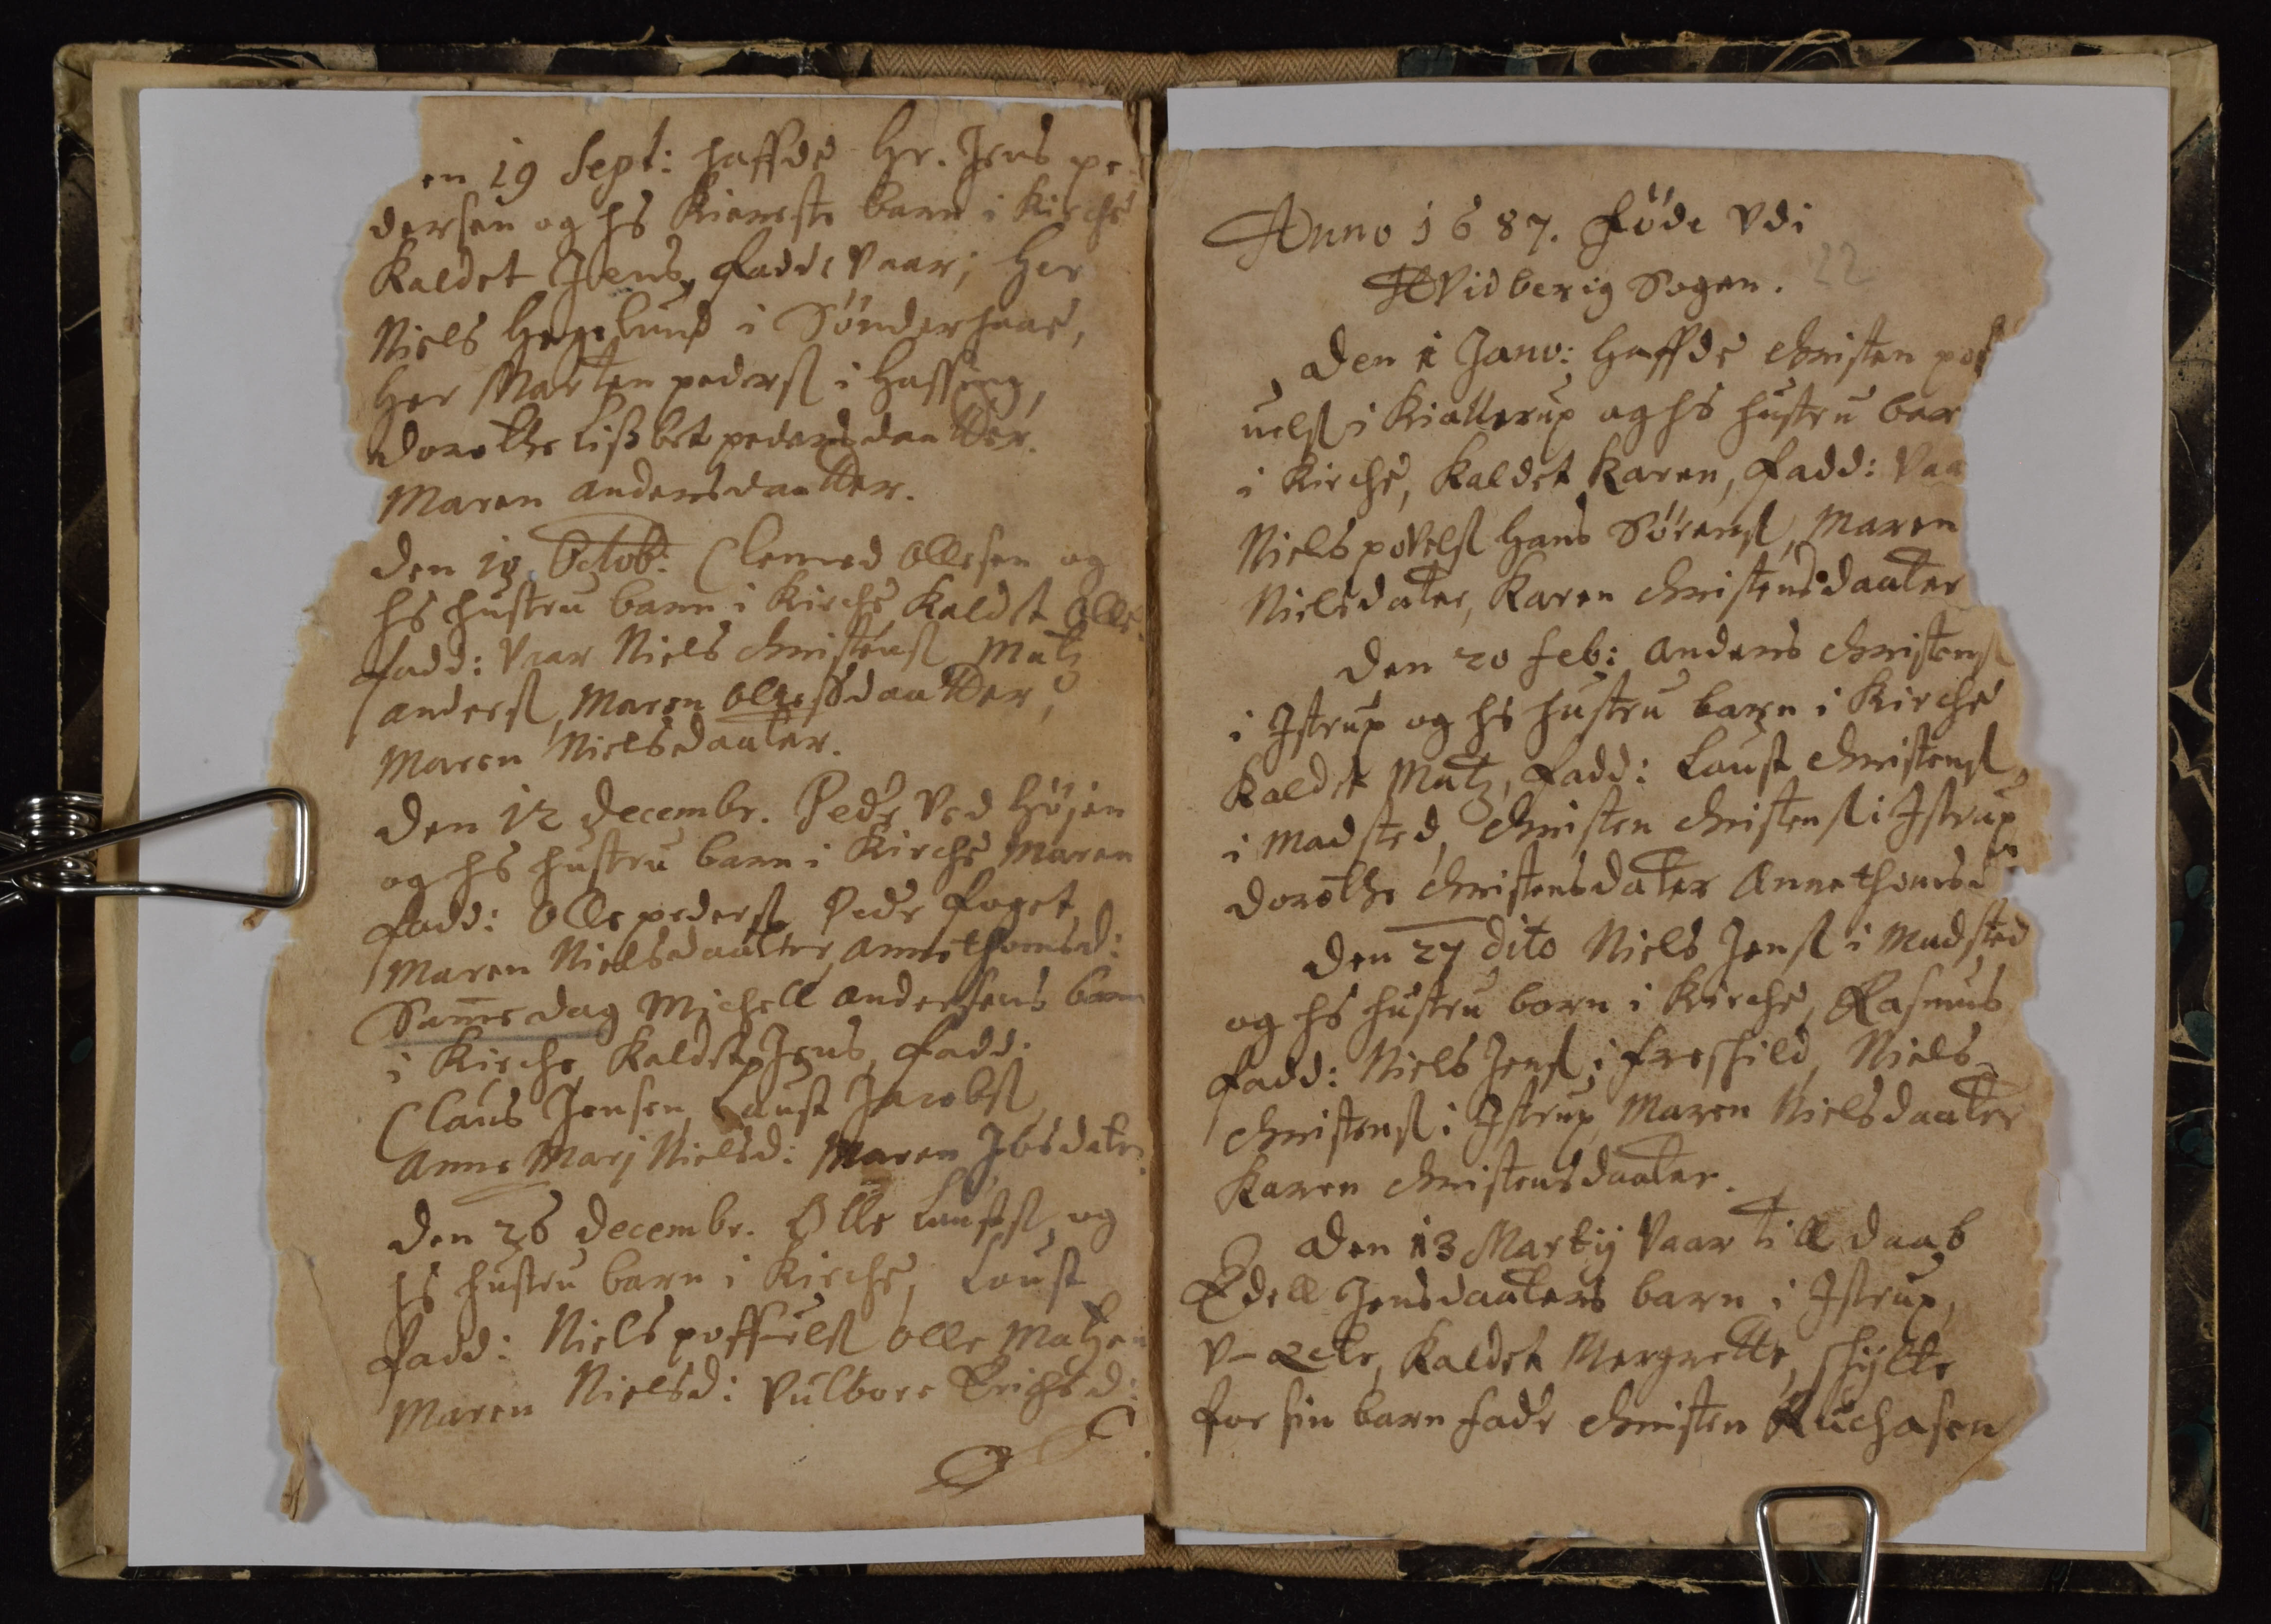en 19 Sept: haffde Hr. Jens pe¬
dersen og hs Kiæreste barn i Kirche
kaldet Jens, Fadd: vaar; Her
Niels Hegelund i Sønderhaae,
Her Marthen Peders i Hassing,
Dorothe Lissbet Pedersdaatter
Maren Andersdaatter
Den 19 Octob: Clemed Ollesen og
hs hustrue barn i Kirche kaldet Olle
Fadd: vaar Niels christens Matz
Anders Maren Ollessdautter,
Maren Nielsdauter.
Den 12 Decembr. Pedr Ved Højen
og hs hustru barn i Kirche Maren
Fsdd: Olle Peders Pedr Foget
Maren Nielsdautter Anne thomsd:
Samme dag Michell Andersens barn
i Kirche kaldet Jens, Fadd.
Claus Jensen Laust Jacobs
Anne Marj Nielsd: Maren Ibsdater
Den 26 Decembr. Olle Lausts og
hs hustru barn i Kirche, Laust
Fadd: Niels poffuels Olle Matzen
Maren Nielsd: Vulbore Erichsd:
Anno 1687. Føde vdi
Hvidberig Sogen.
Den 1 Janv: Haffde Christen pof
uels i Kiallerup og hs hustru baar
i Kirche, kaldet Karen, Fadde: vaa
Niels povels Hans Sørens, Maren
Nielsdater, Karen Christensdaater
Den 20 Feb: Anders Christens
i Istrup og hs hustru baren i Kirche
kaldet Matz, Fadd: Laust christens
i Madsted, Christen Christens i Istrup
Dorothe Christensdater Anne thomsd
den 27 dito Niels Jens i Madsted
og hs hustru barn i Kirche, Rasmus
Fadd: Niels Jens i Freshild, Niels
Christens i Istrup, Maren Nielsdaater
Karen christensdaater.
Den 13 Martijy vaar till daab
Edell Jensdaaters barn i Istrup.
V-æcte, kaldet Margrette, shylte
for sin barn fadr christen Luchasen
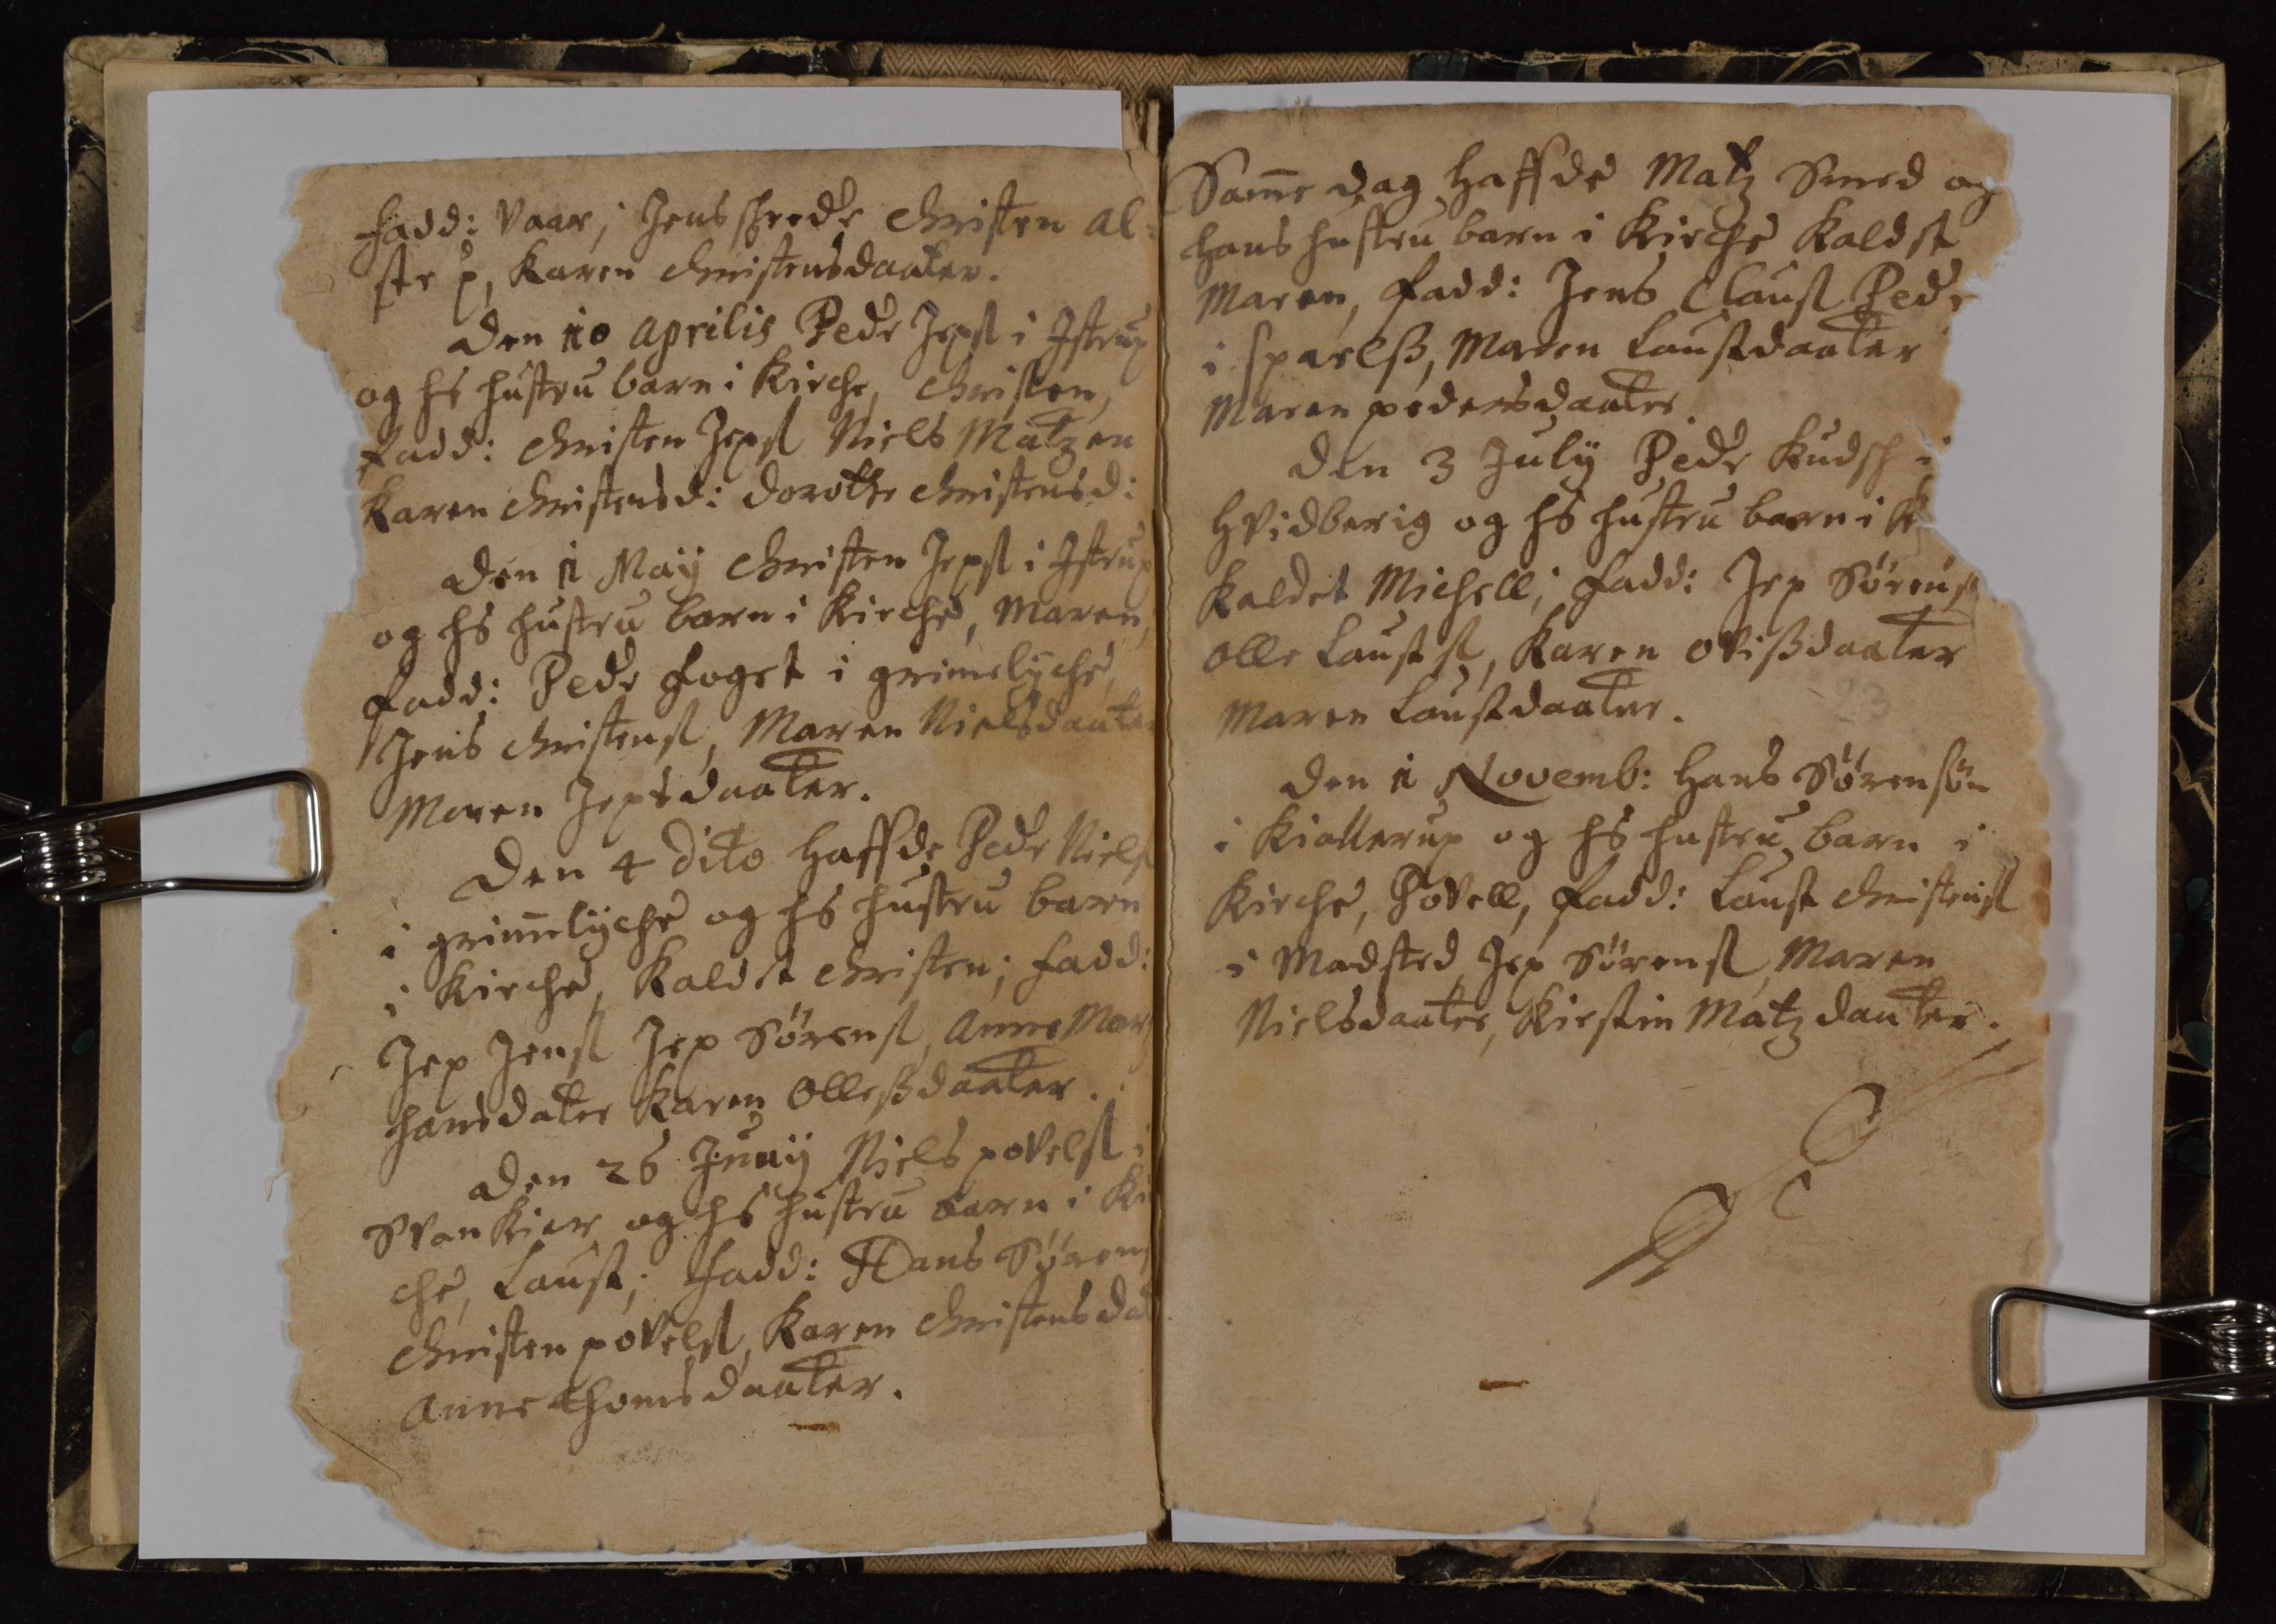Fadd: vaar; Jens shredr Christen Al¬
strup Karen Christensdaater.
Den 10 Aprilis Pedr Jens i Istrup
og hs hustru barn i Kirche, christen
Fadd.: christen Jeps Niels Matzen
Karen Christensd: Dorothe Christensd:
Den 1 Maij christen Jeps i Istrup
og hs hustru barn i Kirche, Maren
Fadd: Pedr Foget i grimelyche
Jens Christens, Maren Nielsdaater
Maren Jepsdaater.
den 4 Dito Haffde Pedr Niels
i Grimmelyche og hs hustru barn
i Kirche, kaldet christen; Fadde:
Jep Jens Jep Sørens Anne Mar
hansdater Karen Ollesdaater
den 26 Junij Niels povels i
Svankier og hs hustru barn i Ki
che Laust; Fadd: Hans Sørens
christen povels, Karen christens dat
Anne thomsdaater.
Samme dag Haffde Matz Smed og
hans hustru barn i Kirche kaldet
Maren, Fadd: Jens Claus Pedr
i Sparlss, Maren Lausdaater
Maren Pedersdaater.
Den 3 Julij Pedr Kudsh i
Hvidberig og hs hustru barn i K
kaldet Michell; Fadd: Jep Sørens
Olle Lausts, Karen Ovissdaater
Maren Lausdaater.
Den 1 Novemb: Hans Sørensøn
Kiallerup og hs hustru barn i
Kirche, Povell, Fadd: Laust christens
i Madsted, Jep Sørens Maren
Nielsdaater, Kirstin Matzdauter